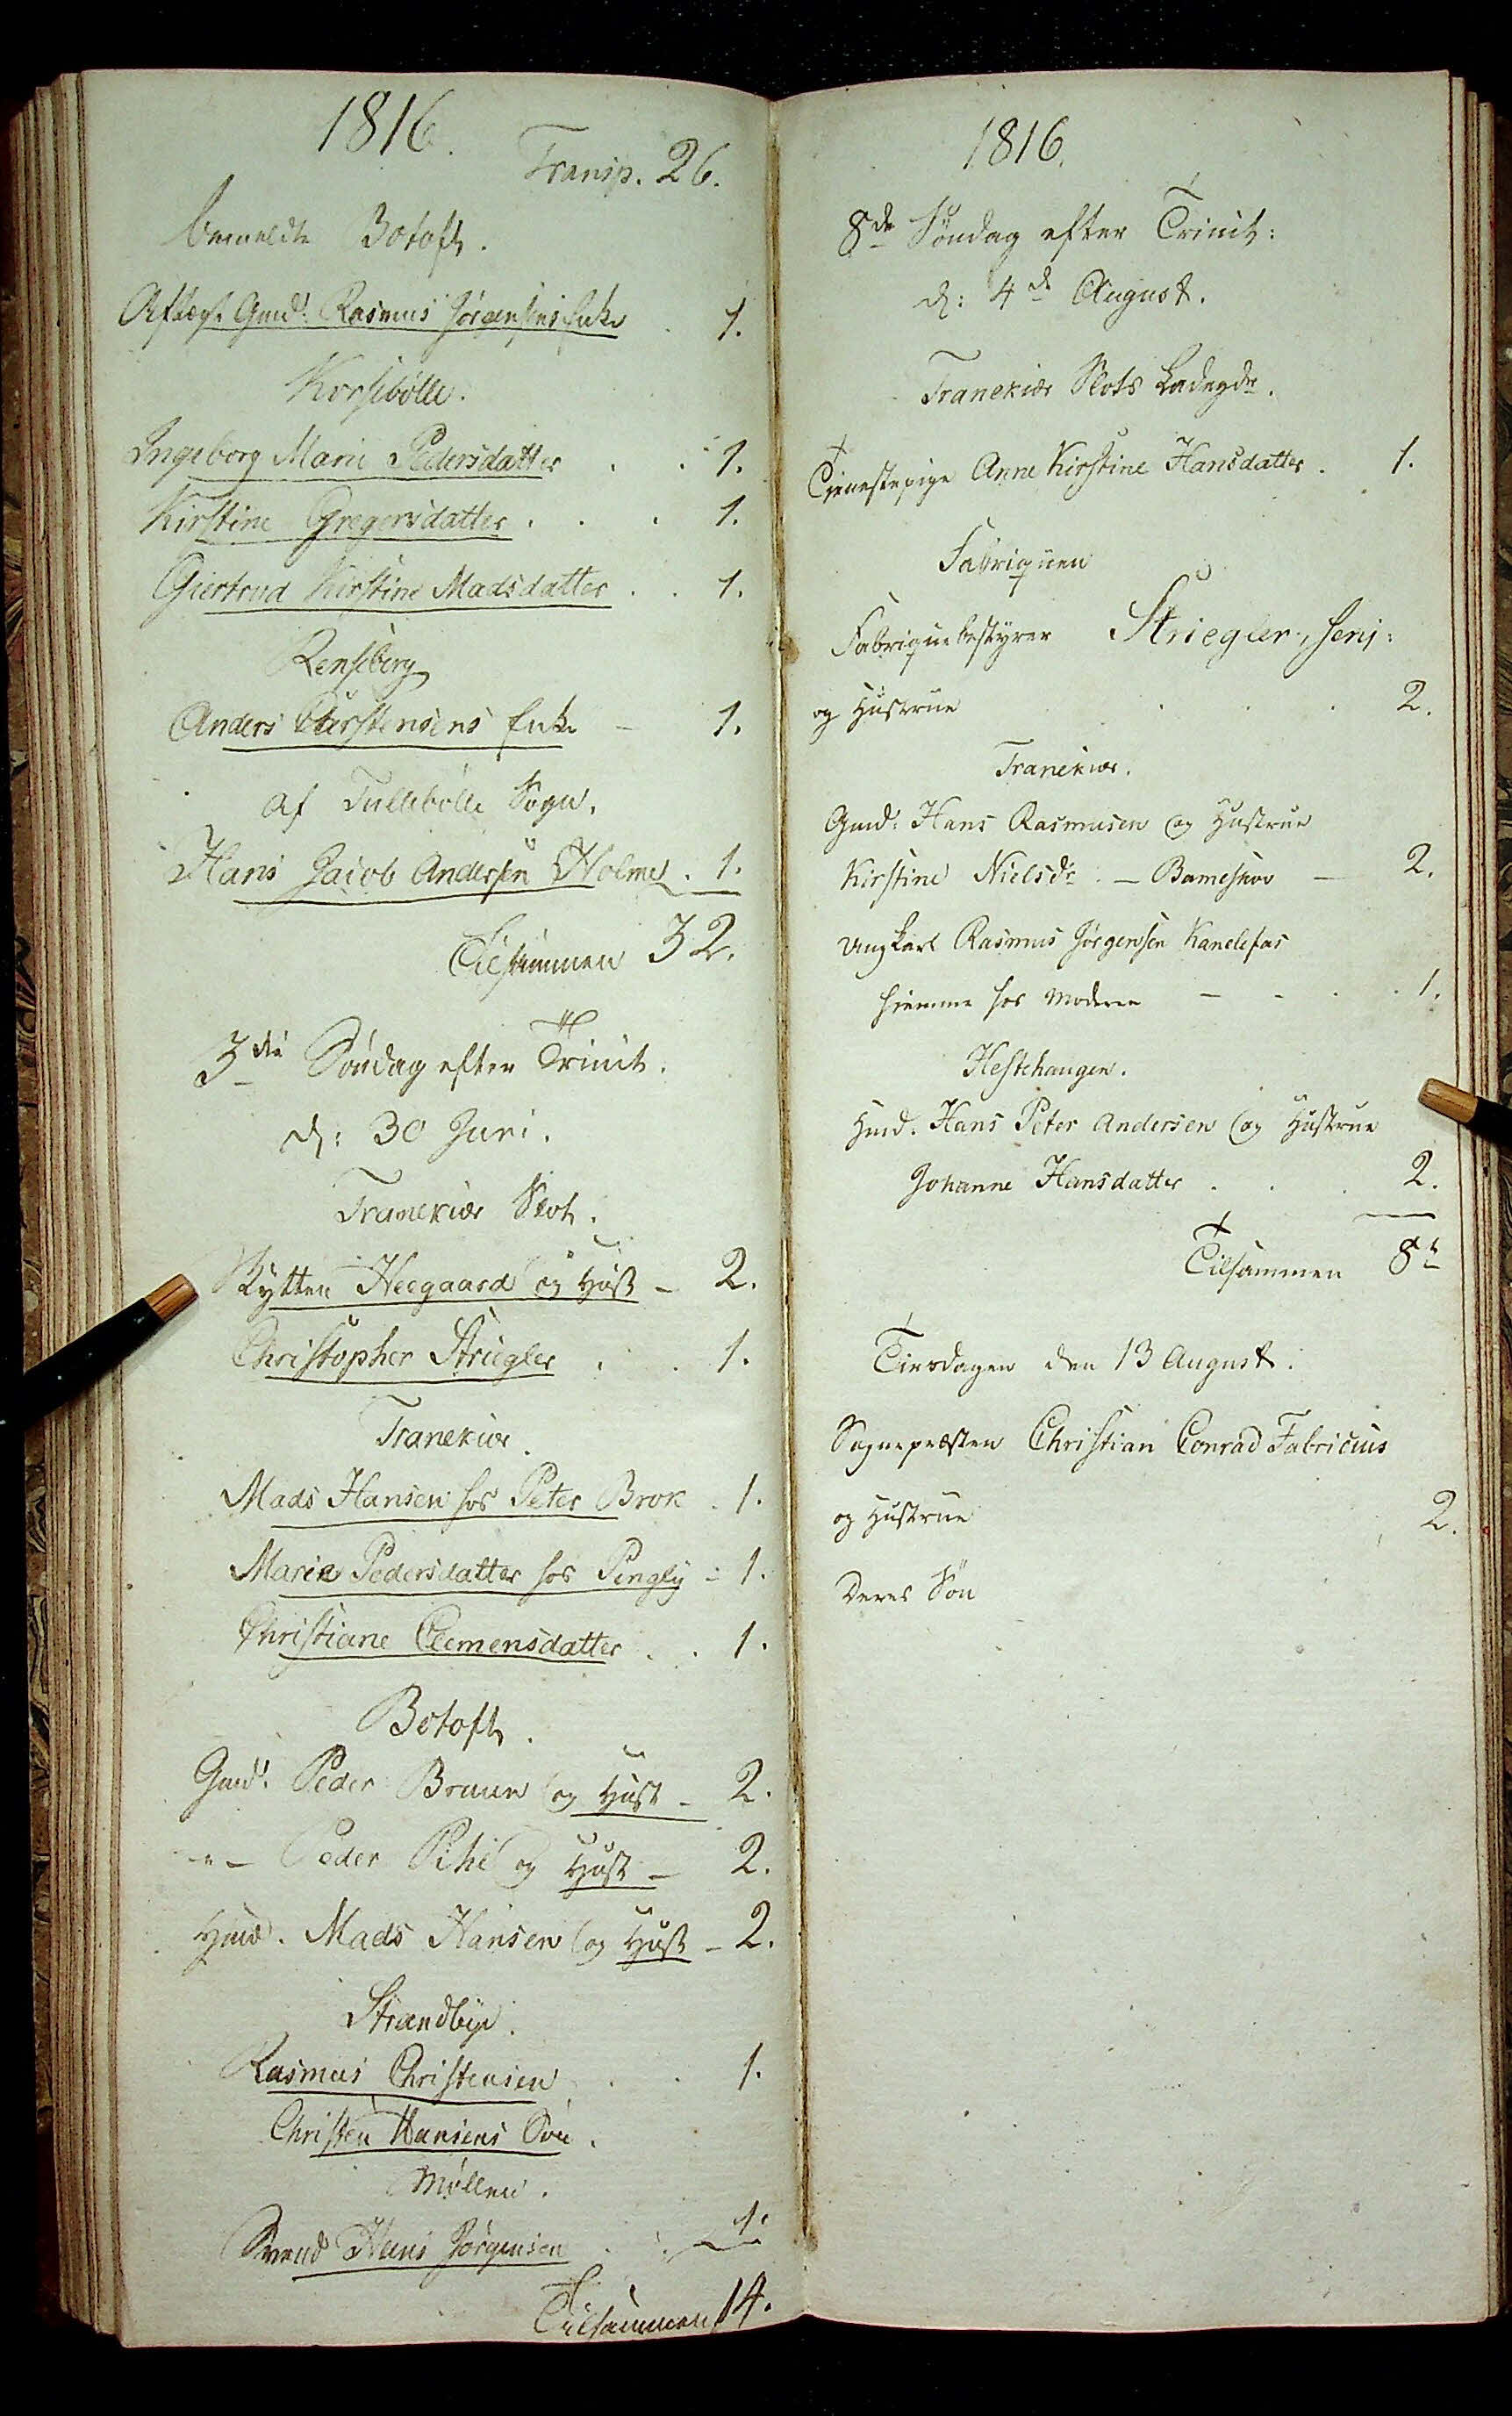1816
Transp. 26.
bemeldte Botoft.
Aftægst Gmd: Rasmus Jørgensens Enke . 1.
Korsebølle.
Ingeborg Marie Pedersdatter . . 1.
Kirstine Gregersdatter . . . 1.
Giertrud Kirstine Madsdatter . . 1.
Renseberg.
Anders Christensens Enke - 1.
af Tullebølle Sogn.
Hans Jacob Andersen Holme . 1.
Tilsammen 32.
3die Søndag efter Trinit.
d: 30 Juni.
Tranekiær Slot.
Skytten Heegaard og Hust - 2.
Christopher Striegler . . 1.
Tranekiær.
Mads Hansen hos Pater Brok . 1
Marie Pedersdatter hos Pingly . 1.
Christiane Clemensdatter . . 1.
Botoft
Gmd. Peder Bruun og Hust - 2
-"- Peder Pihl og Hust - 2.
Hmd. Mads Hansen og Hust - 2.
Strandbye.
Rasmus Christensen . . 1.
Christen Hansens Søn
Møllen.
Smeds Hans Jørgensen . . 1.
Tilsammen 14.
1816.
8de Søndag efter Trinit:
d: 4de August.
Tranekiær Slots Ladegdr.
Tienestepige Anne Kirstine Hansdatter . 1.
Fabriquen
Fabriquebestyrer Striegler, seni:
og Hustrue . . . 2.
Tranekiær.
Gmd: Hans Rasmusen og Hustrue
Kirstine Nielsdr - Bameskov - 2.
Ungkarl Rasmus Jørgensen Kanelefas
hiemme hos Moderen - . . . 1.
Hestehaugen.
Hmd. Hans Peter Andersen og Hustrue
Johanne Hansdatter . . . 2.
Tilsammen 8##
Tirsdagen den 13 August.
Sognepræsten Christian Conrad Fabricius
og Hustrue 2.
Deres Søn
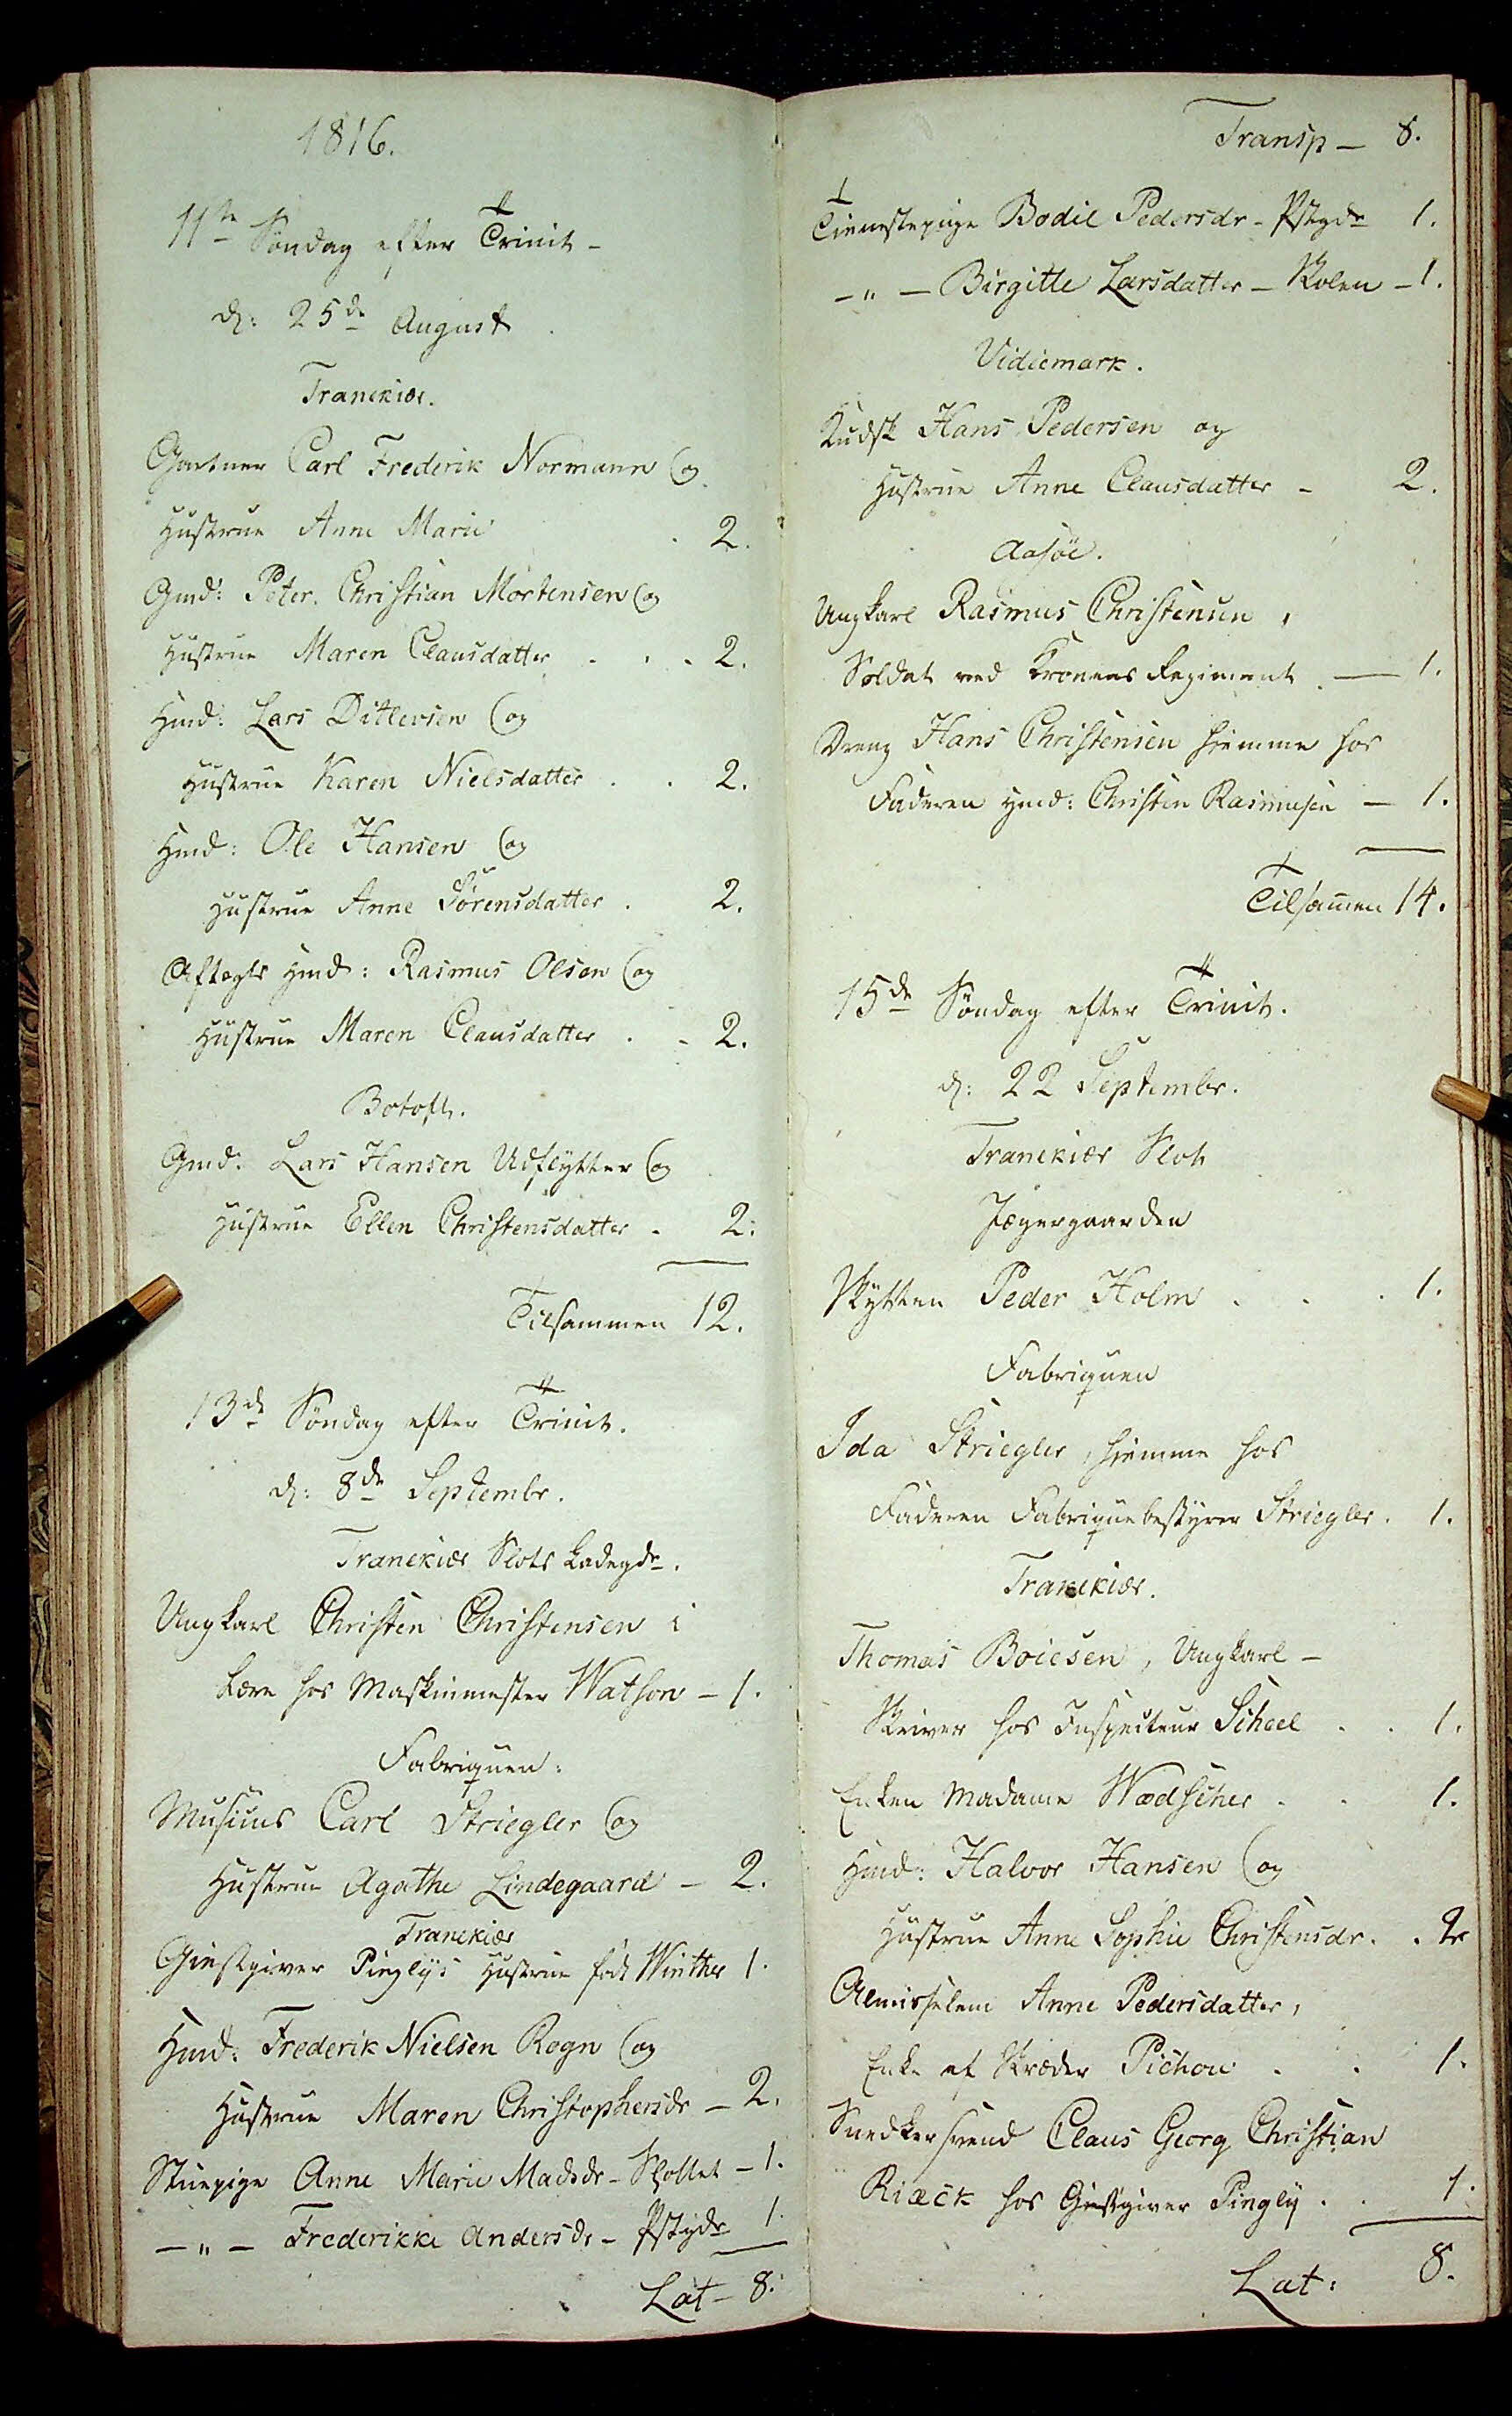1816.
11te Søndag efter Trinit-
d: 25de August
Tranekiær.
Gartner Carl Frederik Normann og
Hustrue Anne Marie . 2.
Gmd: Peter. Christian Mortensen og
Hustrue Maren Clausdatter . . . 2.
Hmd: Lars Ditlevsen og
Hustrue Karen Nielsdatter . . 2.
Hmd: Ole Hansen og
Hustrue Anne Sørensdatter . 2.
Aftægts Hmd: Rasmus Olsen og
Hustrue Maren Clausdatter . .2.
Botoft.
Gmd. Lars Hansen Udlytter og
Hustrue Ellen Christensdatter . 2.
Tilsammen 12.
13de Søndag efter Trinit.
d: 8de Septembr.
Tranekiær Slots Ladegdr.
Ungkarl Christen Christensen i
lære hos Maskinmester Watson - 1
Fabriquen:
Musicus Carl Striegler og
Hustrue Agathe Lindegaard - 2.
Tranekiær
Giestgiver Pinglys Hustrue født Winter 1
Hmd: Frederik Nielsen Rogn og
Hustrue Maren Christophersdr - 2.
Stuepige Anne Marie Madsdr - Slottet - 1.
-"- Frederikke Andersdr - Pstgdr 1
Lat - 8.
Transp - 8.
Tienestepige Bodil Pedersdr Pstgdr 1.
-"- Birgitte Larsdatter - Skolen - 1.
Vidiemark.
Kudsk Hans Pedersen og
Hustrue Anne Clausdatter - 2.
Aasøe.
Ungkarl Rasmus Christensen,
Soldat ved Kronens Regiment - 1.
Dreng Hans Christensen hiemme hos
Faderen Hmd: Christen Rasmusen - 1.
Tilsammen 14.
15de Søndag efter Trinit.
d: 22 Septembr.
Tranekiær Slot
Jægergaarden
Skytten Peder Holm . . . 1.
Fabriquen
Ida Striegler, hiemme hos
Faderen Fabriquebestyrer Striegler . 1.
Tranekiær.
Thomas Boiesen, Ungkarl
Skriver hos Inspecteur Scheel . . 1.
Enken Madame Wædscher . . 1.
Hmd: Halvor Hansen og
Hustrue Anne Sophie Christensdr . . 2.
Almisselem Anne Pedersdatter,
Enke at Skræder Pichou . . 1.
Snedkersvend Claus Gorg Christian
Riæck hos Giestgiver Pingly . . 1.
Lat: 8.